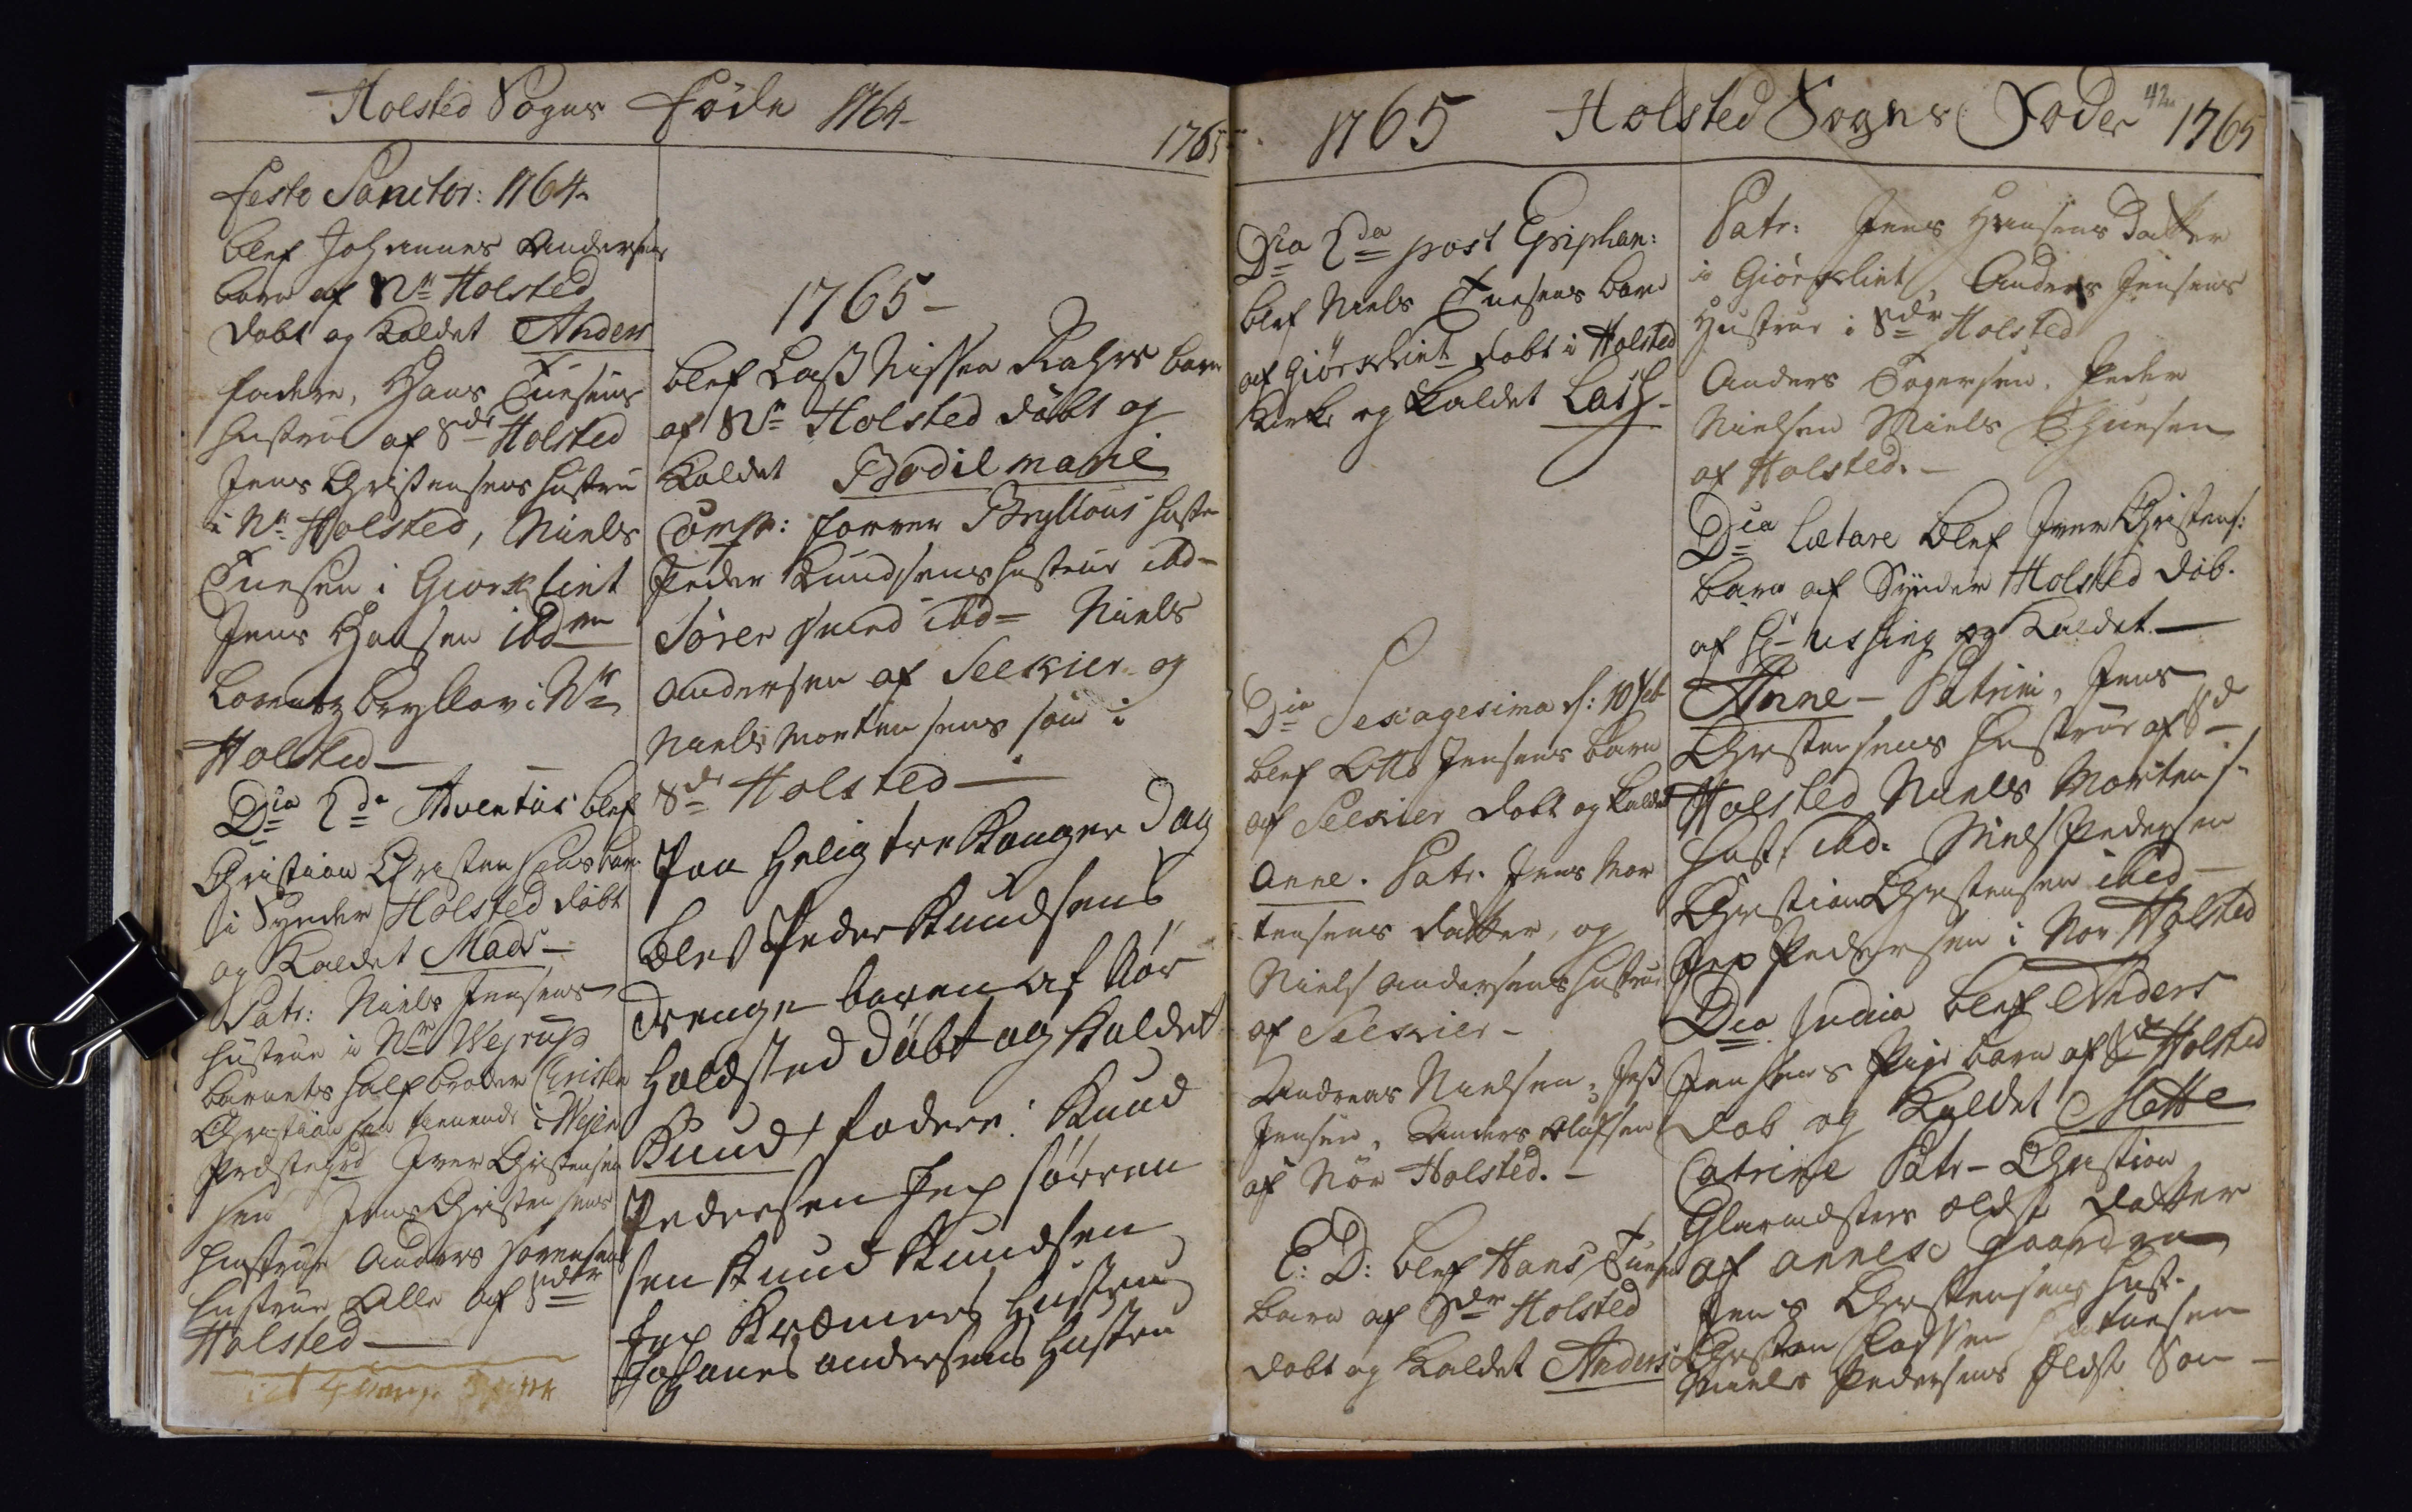Holsted Sogns Føde 1864 -
Festo Sanctor: 1764.
blef Johannes Andersens
barn af Nr Holsted
dobt og kaldet Anders
Fadere, Hans Tuesens
Hustru af Sdr Holsted
Jens Christensens Hustru
i Nr Holsted, Niels
Tuesen i Giørklint
Jens Hansen ibdm
Lorentz Bryllov i Nr
Holsted -
Dca 2de Adventus blef
Christian Christensens Barn
i Synder Holsted døbt
og kaldet Mads -
Patr: Niels Jensens
Hustrue i Nr Wejrup
Barnets half Broder, Christen
Christiansen tienende i Wejen
Præstegrd Iver Christensen
sen Jens Christensens
Hustrue Anders Sørensens
Hustrue, Alle af Sdr
Holsted
i alt 4 drenge 3 piger
1765
1765.
blef Lass Nissen Kahrs barn
af Nr Holsted døbt og
kaldet Bodil marie
Comp: forver Bryllous Hustr
Peder Knudsens Hustrue ibd -
Søren Smed ibd= Niels
Andersen af Seekier og
Niels Mortensens søn i
Sdr Holsted -
Paa Hellig tre Konger Dag
blev Peder Knudsens
Drenge baren af Nør
Holdsted døbt og kaldet
Kund / Fadere Knud
Pedersen Jep Søren
sen Knud Knudsen
Jep Kræmens Hustrue
Johannes Andersens Hustru
Holsted Sogns ##ode
1765
Dca 2da post Epiphan:
blef Niels Tuesens barn
af Giørklint døbt i Holsted
Kirke og kaldet Lass -
Dca Sexagesima d: 10 feb
blef Otto Jensens barn
af Seekier dobt og kaldet
Anne. Patr: Jens Mor
tensens Datter, og
Niels Andersens Hustrue
af Seekier -
Andreas Nielsen ## Jess
Jensen, Anders Olufsen
af Nør Holsted. -
E: D: blef Hans Tues
barn af Sdr Holsted
dobt og kaldet Anders
42
1765
Patr: Jens Hansens Datter
i Giørklint, Anders Jensens
Hustrue i Sdr Holsted
Anders Togersen, Peder
Nielsen Niels Thuesen
af Holsted.
Dca Lætare blef Iver Christens:
barn af Synder Holsted døb.
af Hl - ussing og kaldet -
Anne - Patrini, Jens
Christensens Hustrue af Sdr
Holsted Niels Mortens
Hust: ibd: Niels Pedersen
Christian Christensen ibid -
Jep Pedersen i Nor Holsted
Dca Judica blef Anders
Jensens Pige barn af SdrHolsted
dob og kaldet Mette
Catrine Patr - Christian
Glarmæster ældst Datter
af annesc Gaarden
Jens Christensens Hust.
Christen Lassen ## tuesen
Nieels Pedersens ældst Son
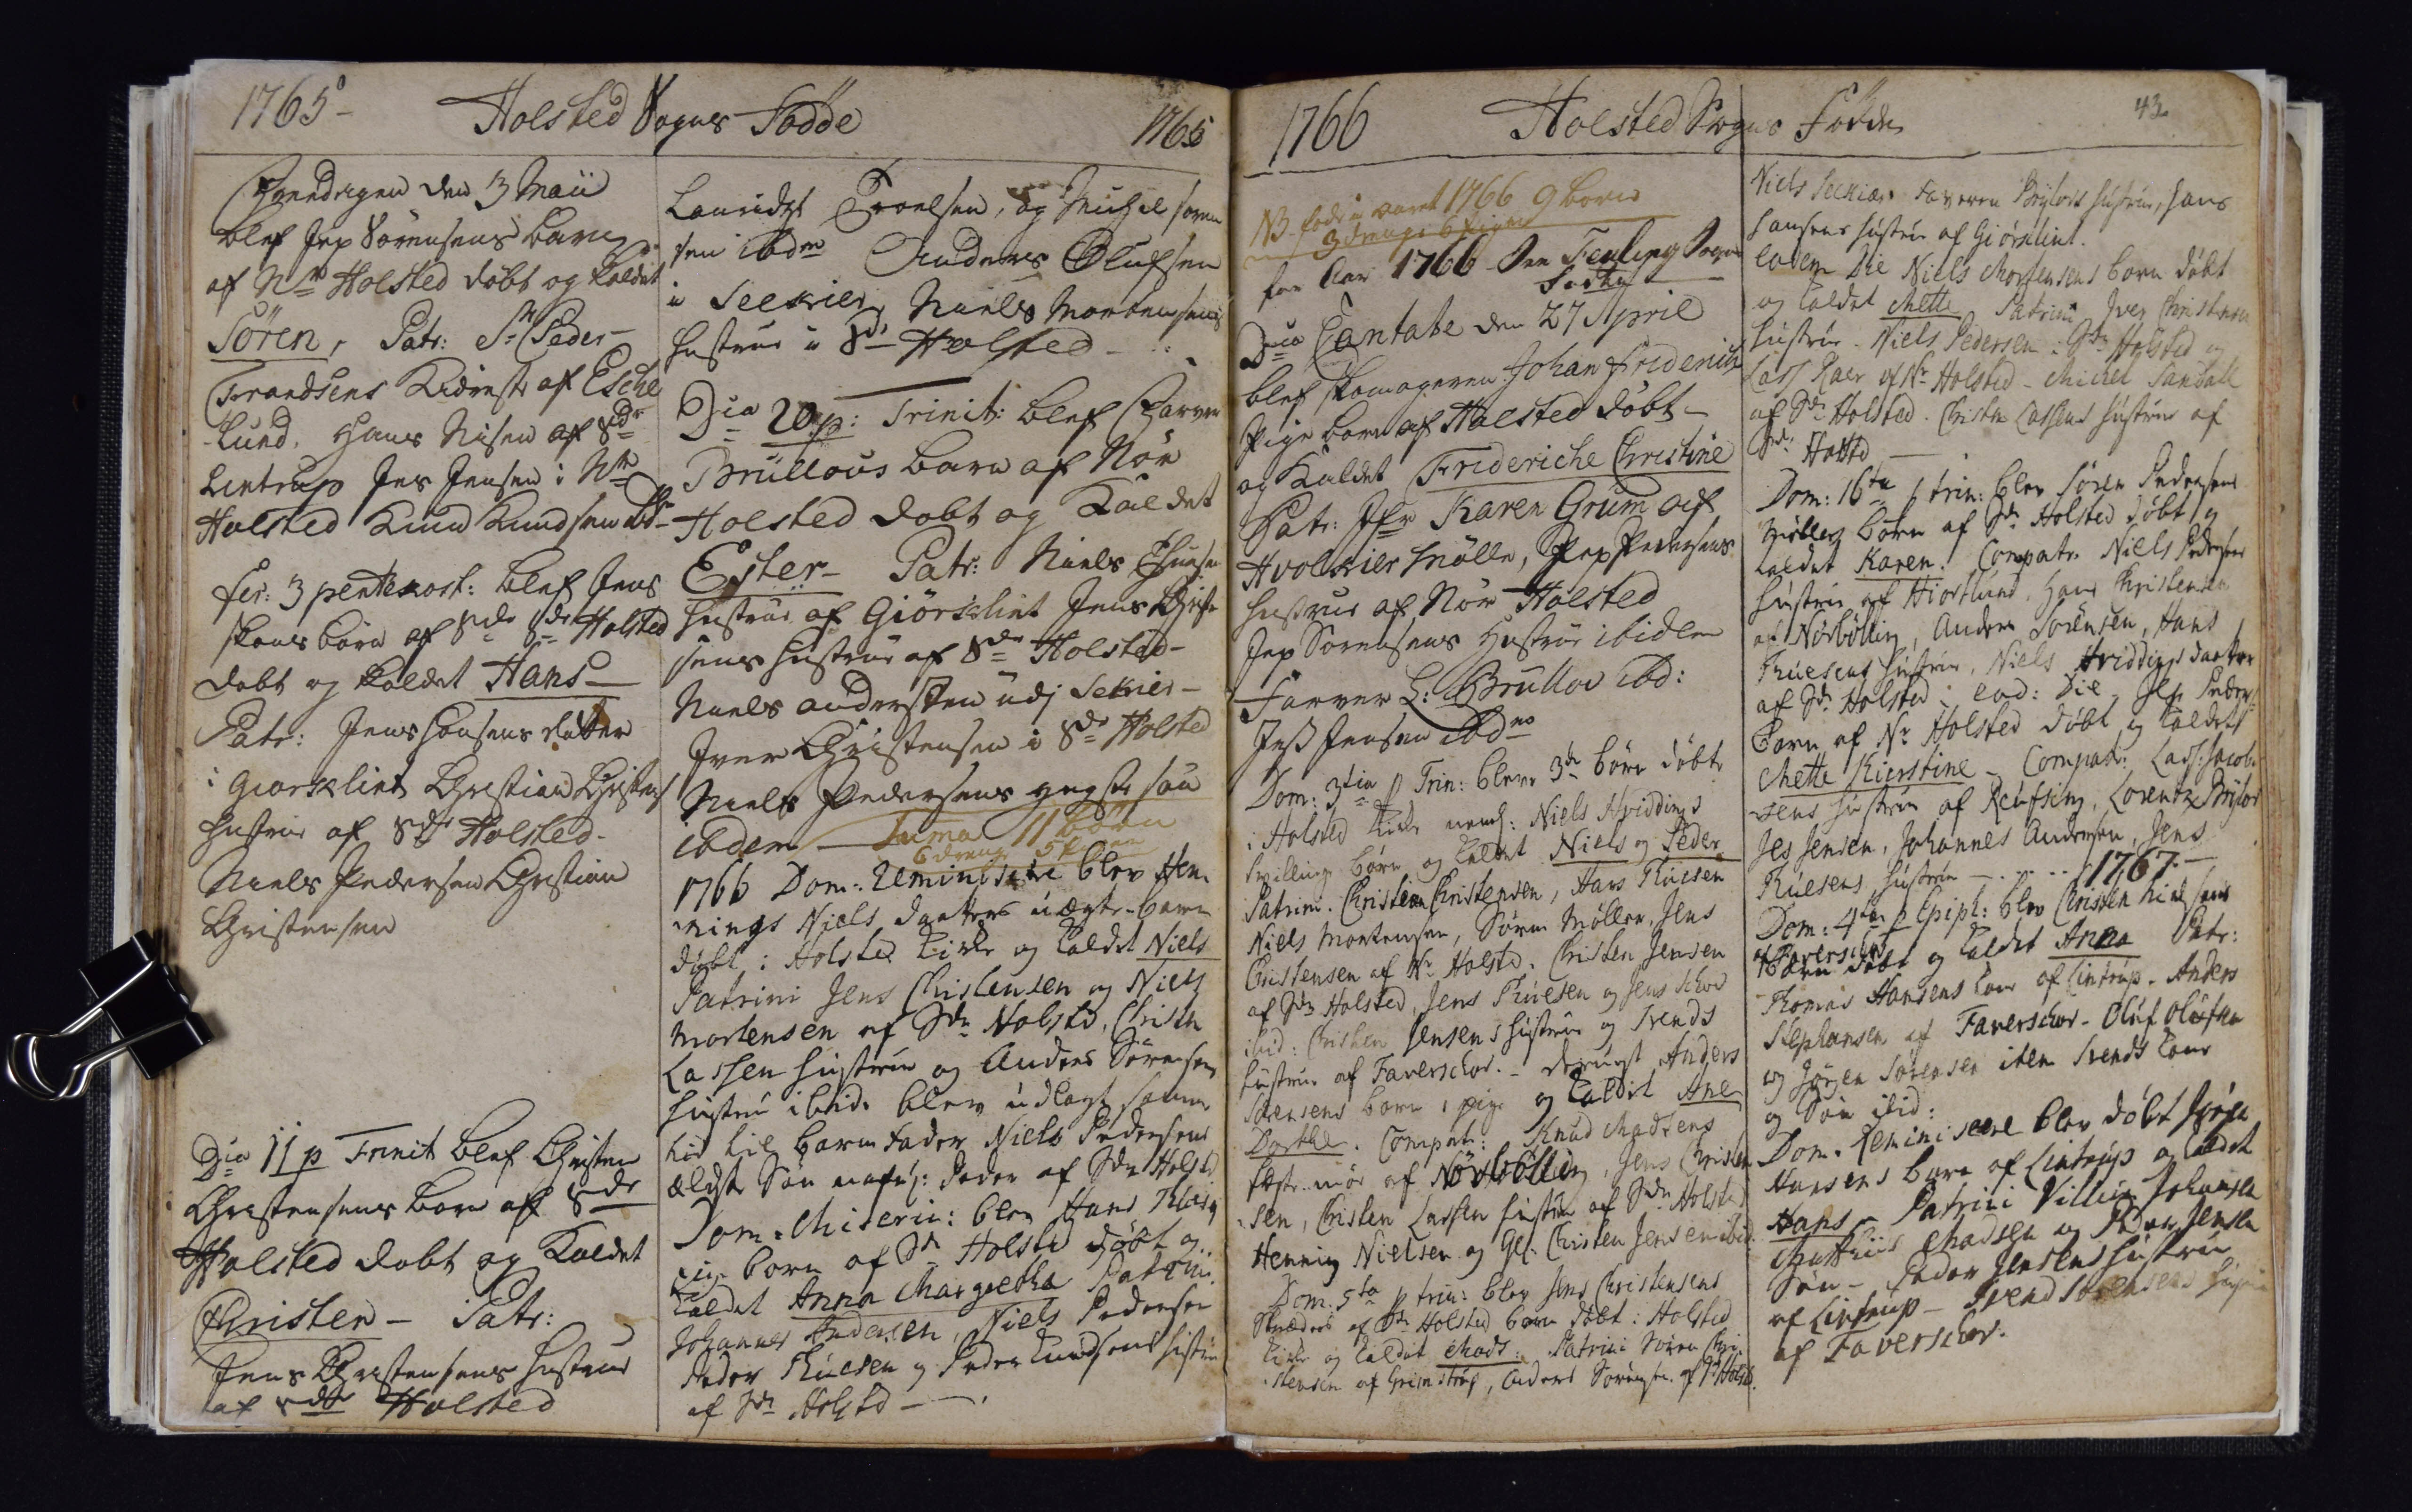1765
Holsted Sogns Fødde
Freedagen den 3 Maii
blef Jep Sørensens barn
af Nr Holsted døbt og kaldet
Søren, Patr: Sr Peder
Frandsens Kiæreste af Esche
Lund, Hans Nissen af Sdr¬
Lintrup Jes Jensen i Nr
Holsted, Knud Knudsen ibdm
Fer: 3 Pentekost: blef Jens
Skous barn af Sdr Holsted
døbt og kaldet Hans -
Patr: Jens Hansens Datter
i Giorklint Christian Christens
Hustrue af Sdr Holsted.
Niels Pedersen Christian
Christensen
Dca 11 p Trinit blef Christen
Christensens barn af Sdr
Holsted dobt og kaldet
Christen - Patr:
Jens Christensens Hustrue
af Sdr Holsted
1765
Lauridz Troelsen, og Michel sorens
sen ibdm Anders Olufsen
i Seekier, Niels Mortensens
Hustrue i Sdr Holsted
Dca 20 p: Trinit: blef Farver
Brullous barn af Nør
Holsted døbt og kaldet
Ester - Patr: Niels Thuesen
Hustrue af Giørklint, Jens Christen
sens Hustrue af Sdr Holsted -
Niels Andersen udi Sekier -
Iver Christensen i Sdr Holsted.
Niels Pedersens yngste søn
ibdem -  Summa 11 Børn
6 dreng 5 piger
1766 Dom: Reminisere blev Hen¬
##ings Niels daatters uægte-barn
døbt i Holsted Kirke og kaldet Niels
Patrini Jens Christensen og Niels
Mortensen af Sdr Holsted, Christen
Lassen Hustrue og Anders Sørensen
Hustru ibid: blev udlagt samme
tid til barne Fader Niels Pedersens
ældste Søn nafnl: Peder af Sdr Holsted
Dom: Miseric: blev Hans Thuesens
pige barn af Sdr Holsted døbt og
kaldet Anna Margretha Patrini
Johannes Andersen, Niels Pedersen
Peder Thuesen og Peder Knudsens Hustru
af Sdr Holstd -
Holsted Sogns Fødde
1766
NB. fode i aaret 1766 9 børn
3 drenge 6 piger
for Aar 1766. ## Feuling Sogns
fodte
Dca Cantate den 27 April
blev Skomageren Johan Friderichs
Pige barn af Holsted døbt -
og kaldet Frideriche Christine
Patr: Jfr Karen Grum af
Hvolkier Mølle, Jep Pedersens
Hustrue af Nør Holsted.
Jep Sørensens Hustrue ibidem
Farver L: Brullov ibd:
Jess Jensen ibdm
Dom: 3tia p Trin: blev 3de børn døbt
i Holsted Kirke navnl: Niels Hviddings
tvillinge børn og kaldet Niels og Peder
Patrini. Christen Christensen, Hans Thuesen
Niels Mortensen, Søren Møller, Jens
Christensen af Nr Holsted, Christen Jensen
af Sdr Holsted, Jens Thuesen og Jens Schov
ibid: Christen Jensens Hustrue og Svends
Hustrue af Faverschov. - dernest Anders
Sørensens barn 1 pige og kaldet Ane
Dorthe. Compat: Knud Madsens
Fæstemøe af Nørbølling, Jens Christen
sen, Christen Larsens Hustru af Sdr Holsted
Henning Nielsen og Gl: Christen Jensen ibid
Dom: 5ta p Trin: blev Jens Christensens
Skræders af Sdr Holsted barn døbt i Holsted
Kirke og kaldet Mads: Patrini Søren Chri¬
stensen af Grimstrup, Anders Sørensen af Sdr Holsted
Niels Seekiær. Farver Brylovs Hustrue, Hans
Hansens Hustrue af Giørklint.
Eodem Die Niels Mortensens barn døbt
og kaldet Mette Patrini Iver Christensens
Hustrue, Niels Pedersen i Sdr Holsted og
Lass Ka##r af Nr Holsted Michel Sandall
af Sdr Holsted. Christen Lassens Hustrue af
Sdr Holsted
Dom: 16ta p Trin: blev Søren Pedersen
Mølles barn af Sdr Holsted døbt og
kaldet Karen. Compatr. Niels Pedersens
Hustrue af Hiortlund, Hans Christensen
af Norbølling, Anders Sørensen, Hans
Thuesens Hustru, Niels Hviddings daatter
af Sdr Holsted eod: Die: Jep Peders:
Barn af Nr Holsted døbt og kaldet
Mette Kierstine Compatr: Lass: Jacob
sens Hustru af Reusing, Lorentz Brylov
Jes Jensen, Johannes Andersen, Jens
Thuesens Hustrue.
1767
Dom: 4## p Epiph: blev Christen Nielsens
af Faverschov
barn døbt og kaldet Anne Patr:
Thomas Hansens Kone af Lintrup. Anders
Stephansen af Faverschov. Oluf Olufsen
og Jørgen Sørensen item Svends Kone
og Søn ibid:
Dom. Remini ## blev døbt Jørgen
Hansens Barn af Lintrup og kaldet
Hans Patrini Villum Johansen
Mattiis Madsen og Peder Jensen
Søn - Peder Jensens Hustru
af Lintrup= Svend Sørensens Hustrue
af Faverschov.